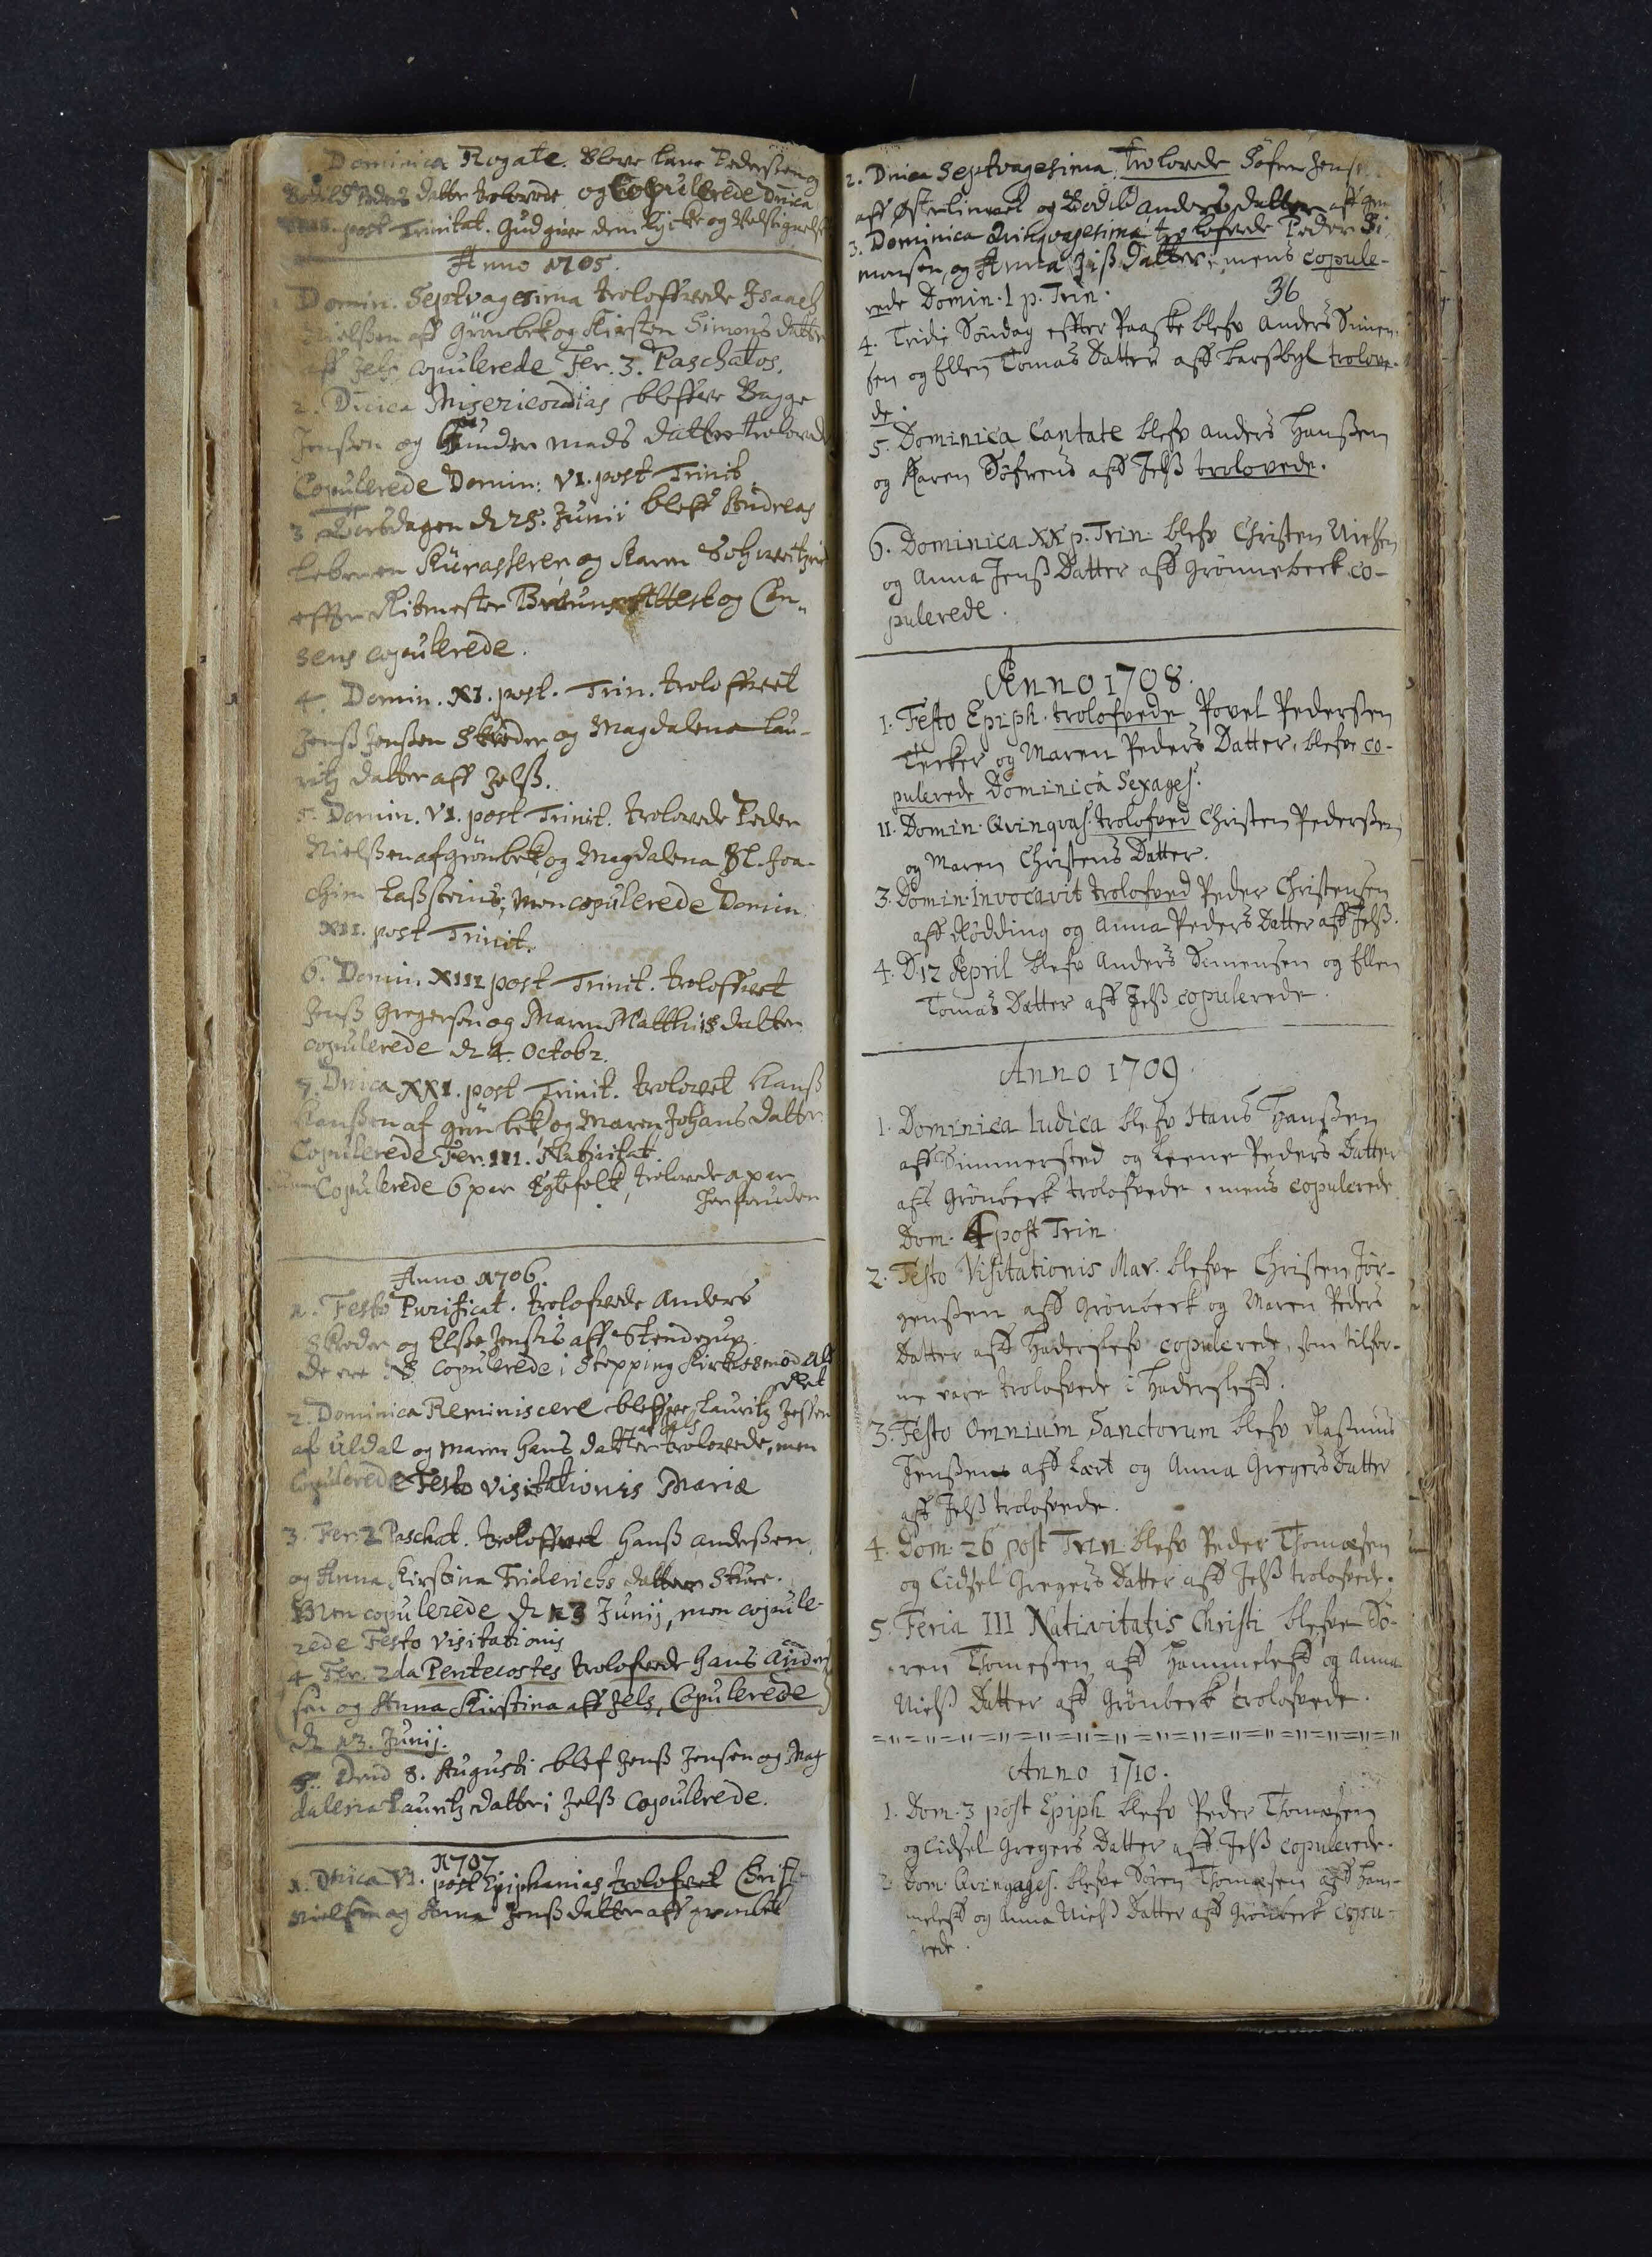Dominica Rogate bleve Laue Pederssen og
Bodild Peders Datter trolovede og copulerede Dnica
post Trinitat. Gud give dem Lycke og Velsignelse.
Anno 1705
1. Domin. Septvagesimo troloffvede Isaach
Nielssen aff Grønbek og Kirsten Simons Datter
aff Jels. copulerede Fer. 3. Paschatos.
2. Dnica Misericordias bleffve Bagge
Jenssen og Gunder Mads Datter trolovede
Copulerede Domin: VI post Trinit.
3. Torsdagen d 25. Junii bleff Andreas
Leben en Kurasserer og Karen Schveitzer
effter Ritmester Bruns Attest og Con¬
sens copulerede
4. Domin. XI. post Trin. trolofvet
Jenss Jenssen Skreder og Magdalena Lau¬
ritz Datter aff Jelss.
5. Domin. VI. post Trinit. trolovede Peder
Nielssen af Grønbek og Magdalena Sl. Joa¬
chim Lassteins, men copulerede Domin
XII post Trinit. 
6. Domin. XIII post Trinit. trolofvet
Jenss Gregersen og Maren Matthis Datter.
copulerede d 4. Octobr
7. Dnica XXI. post Trinit. trolovet Hanss
Hanssen af Grønbek og Maren Johans Datter
Copulerede Fer. III. Nativitat.
Copulerede 6 par Egtefolk, trolovede 1 par
her foruden
Anno 1706
1. Festo Purificat. trolofvede Anders
Skreder og Elsse Jensis aff Stenderup
De ere NB copulerede i Stepping Kirke mod
##et
2. Dominica Reminiscere bleffve Lauritz Jessen
af Jels
af Uldal og Maren Hans Datter trolovede, men
Copulerede Festo Visitationis Mariæ
3. Fer. 2 Paschat. troloffvet Hanss Anderssen
og Anna Kirstina Friderichs Datter Stuer.
Blev copulerede d 13 Junij, men copule¬
rede Festo Visitationis
4. Fer. 2da Pentecostes trolofvede Hans Ander
sen og Anna Kirstina aff Jels, Copulerede
d 13. Junij.
5. Dend 8. Augusti blef Jenss Jensen og Mag
dalena Lauritz Datter i Jelss copulerede.
1707.
1. Dnica VI. Post Epiphanias trolofvet Christ 
Nielsen og Anna Jenss Datter aff grønbek
36
2. Dnica Septvagesima trolovede Søfren Jens 
aff Østerlinnet og Bodild Anders Datter aff
3. Dominica Quinqvagesima trolofvede Peder Si¬
monsen, og Anna Jiss Datter, mens copule¬
rede Domin. 1 p. Trin
4. Tridie Søndag effter Paaske blefv Anders Simen¬
sen og Ellen Tomas Datter aff Barsbyl trolove¬
de.
5. Dominica Cantate blef Anders Hanssen
og Karen Søfrens aff Jelss trolovede.
6. Dominica XX p. Trin. blefv Christen Nielsen,
og Anna Jenss Datter aff Grønnebeck co¬
pulerede
Anno 1708.
I. Festo Epiph. trolofvede Povel Pedersen
Tecker og Maren Peders Datter, blefv co¬
pulerede Dominica Sexages.
II. Domin. Qvinqvas. trolofved Christen Pederssen
og Maren Christens Datter
3. Domin. Invocavit trolofved Peder Christensen
aff Rødding og Anna Peders Datter aff Jelss.
4. D 12 April blefv Anders Simensen og Ellen
Tomas Datter aff Jelss copulerede
Anno 1709
1. Dominica Iudica blefv Hans Hanssen
aff Simmersted og Leene Peders Datter 
aff Grønbeck trolofvede, mens copulerede
Dom. 4 post Trin
2. Festo Visitationis Mar. Blefve Christen Jør¬
gensen aff Grønbeck og Maren Peders
Datter aff Haderslefv copulerede, som tilfor¬
ne var trolofvede i Hadersleff.
3. Festo Omnium Sanctorum blefv Rasmus
Jenssen aff Lart og Anna Gregers Datter
aff Jelss trolofvede.
4. Dom. 26 post Trin. blefv Peder Thomasen
og Cidsel Gregers Datter aff Jelss trolofvede.
5. Feria III Nativitatis Christi blefve Sø¬
ren Thomesen aff Hammeleff og Anna
Niels Datter aff Grønbeck trolofvede
Anno 1710
1. Dom. 3 post Epiph blefv Peder Thomasen
og Cidsel Gregers Datter aff Jelss copulerede.
2. Dom. Qvinqages. blefve Søren Thomasen aff Ham¬
meleff og Anna Niels Datter aff Grønbeck copu¬
ede